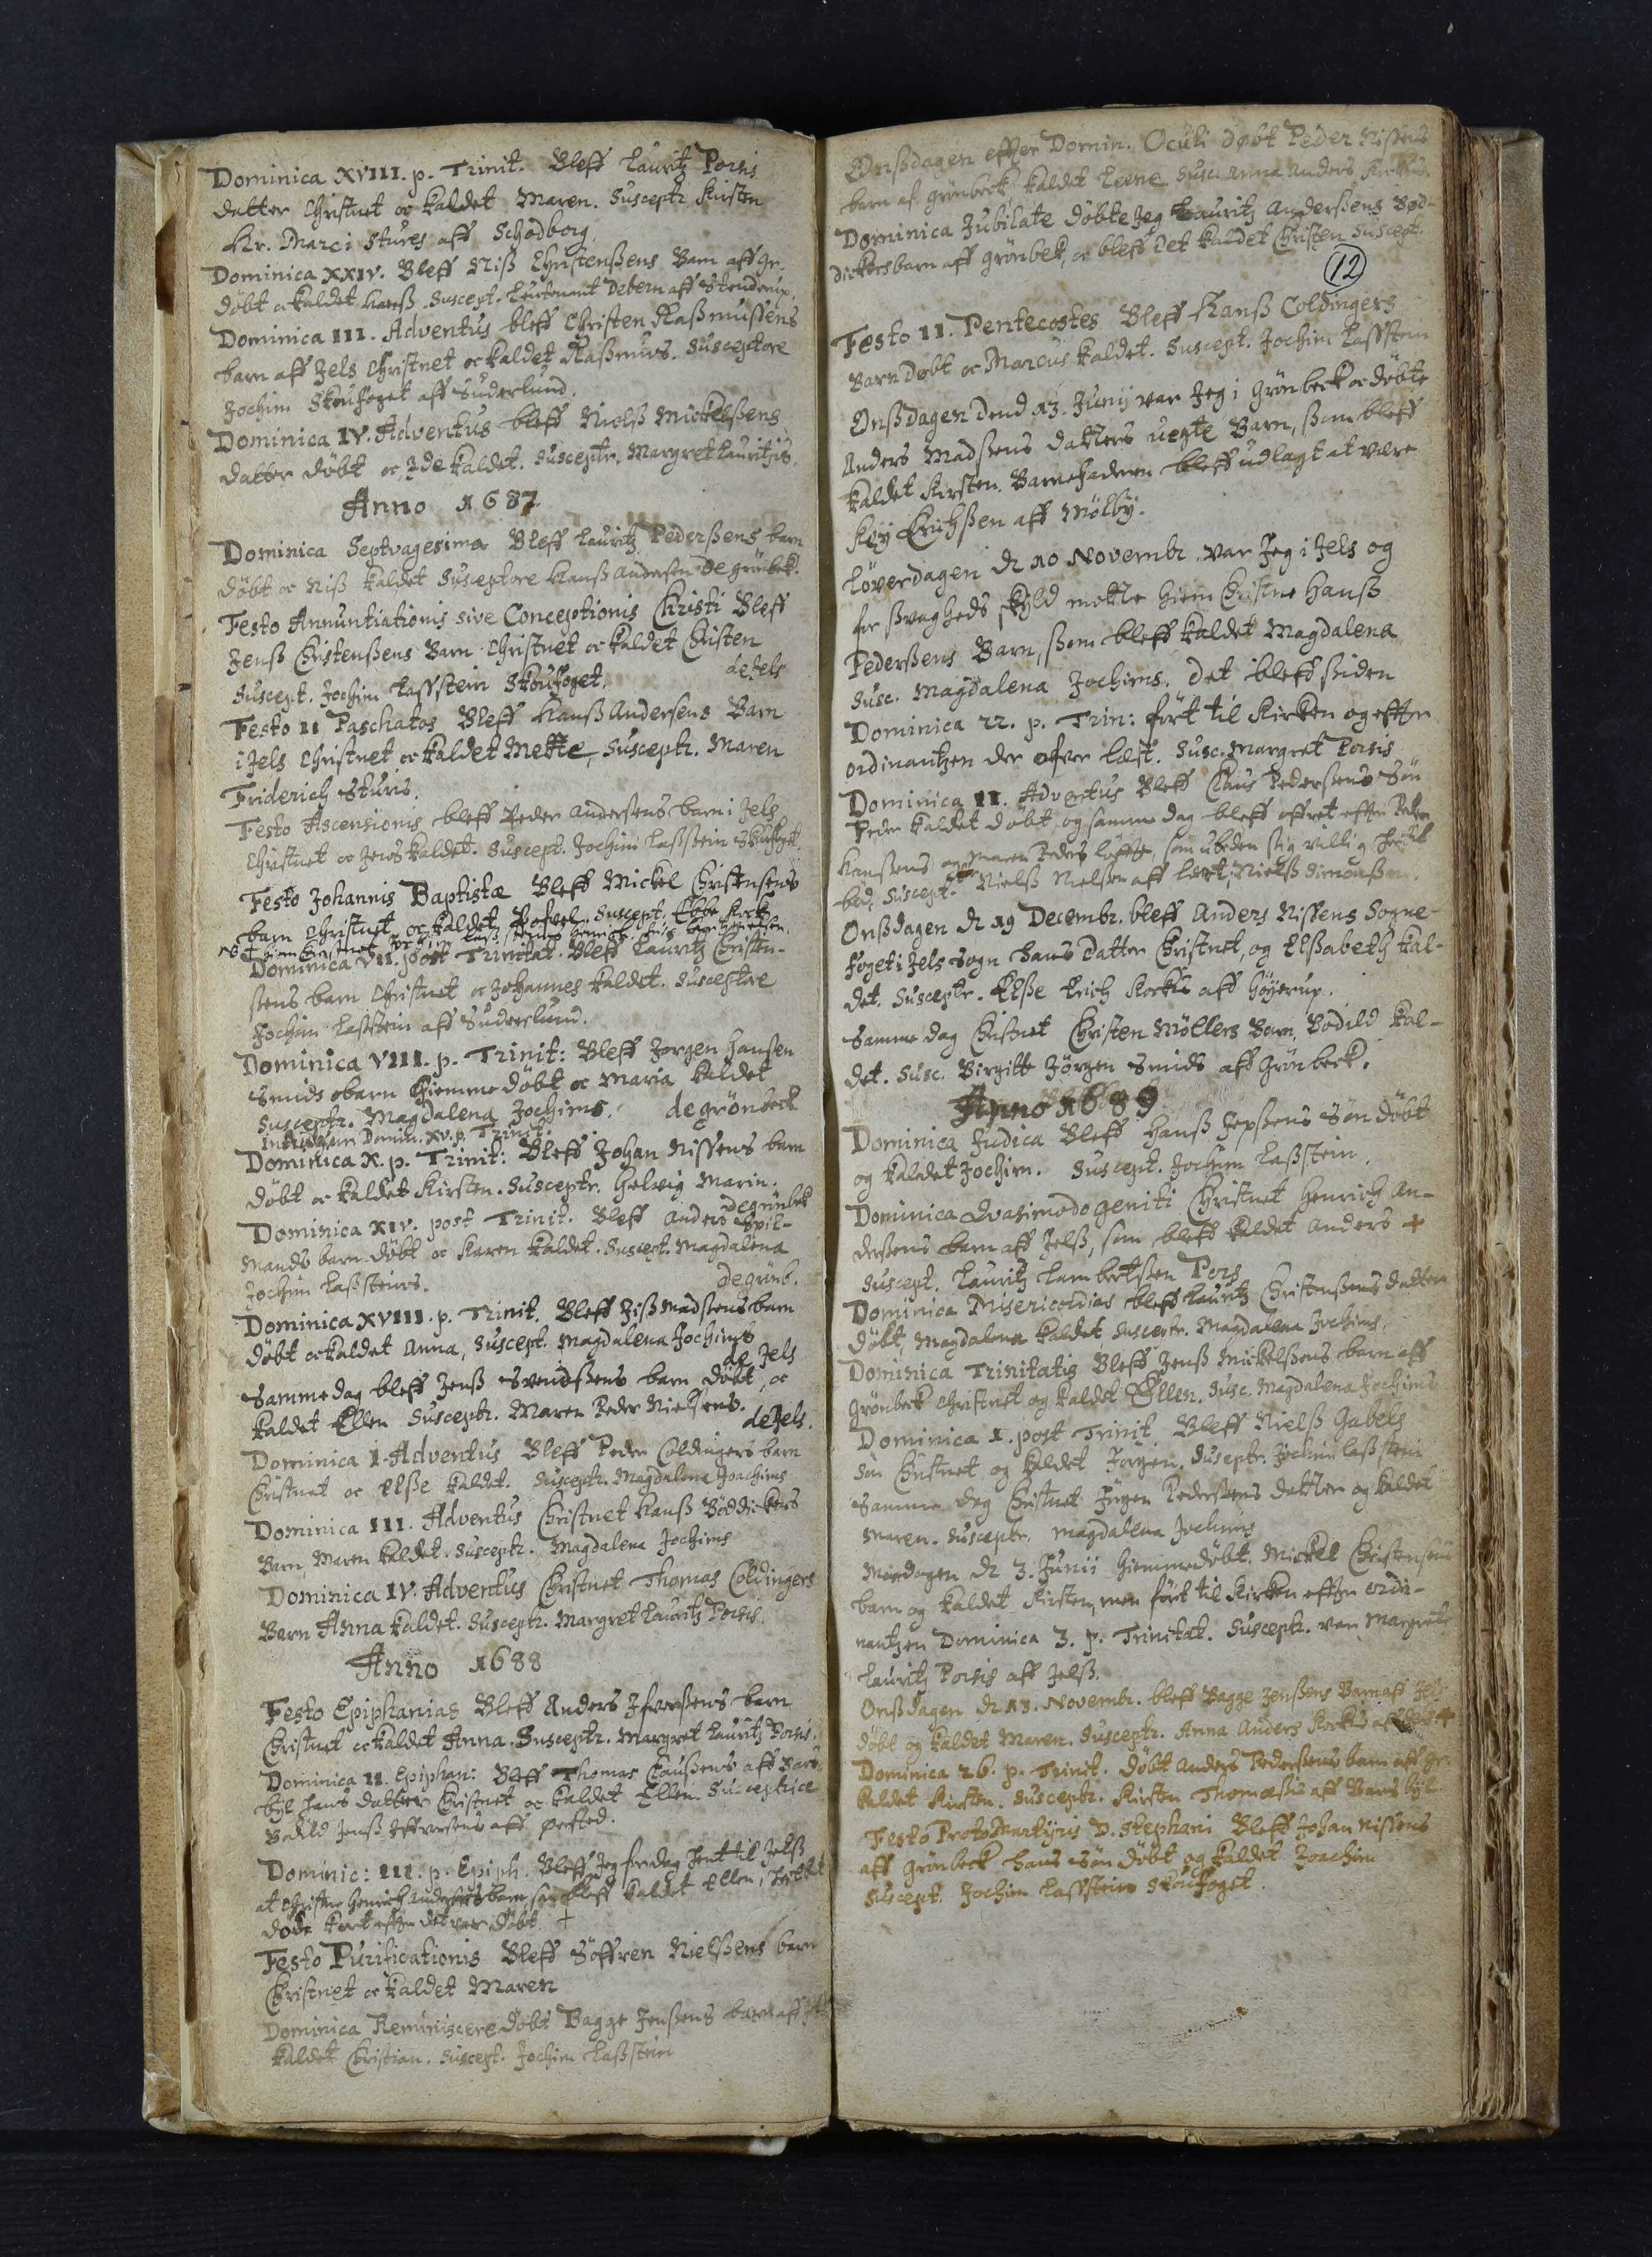Dominica XVIII. p. Trinit. Bleff Lauritz Porsis
datter christnet oc kaldet Maren. Susceptr Kirsten 
HR. Marci Stures aff Schodborg.
Dominica XXIV. Bleff Niss Christensens Barn aff Gr.
døbt oc kaldet Hanss Suscept. Leutnant Debern aff Stenderup.
Dominica III. Adventus bleff Christen Rasmussens
barn aff Jels christnet oc kaldet Rasmus. Susceptore
Jochim Skoufoget aff Suderlund.
Dominica IV. Adventus bleff Niels Mickelssens
datter døbt oc Ide kaldet. Susceptr. Mergret Lauritzis.
Anno 1687.
Dominica Septvagesima Bleff Lauritz Pedersens barn
døbt oc Niss kaldet Susceptore Hans Andersen de Grønbeck.
Festo Annuntiations sive Concesotionis Christi Bleff
Jens Christensens Barn christnet oc kaldet Cristen.
Suscept. Jochim Lasstein Skoufoget.
de Jels
Festo II Paschatos Bleff Hans Andersens Barn
i Jels christnet oc kaldet Mette. Susceptr. Maren
Friderich Sturis.
Festo Ascensionis bleff Peder Andersens barn i Jels
christnet oc Jens kaldet. Suscept. Jochim Lasstein Skoufoget.
Festo Johannis Baptistæ Bleff Mickel Christensens
barn christnet oc kaldet Pofvel. Suscept. Ebbe Kock.
NB + hiemchristnet Jochim Lasstein Gr## ## ## ## ##
Dominica VII post Trinitat. Bleff Lauritz Christen¬
sens barn christnet oc Johannes kaldet. Susceptore
Jochim Lassteins aff Suderlund.
Dominica VIII p. Trinit: Bleff Jørgen Hansen
Smids barn Hiemme Døbt oc Maria kaldet
Susceptr. Magdalena Jochims. de Grønbeck.
in## Domin. XV. p. Trinit.
Dominica X. p. Trinit: Bleff Johan Nissens barn
døbt oc kaldet Kirsten. Susceptr. Helvig Marin.
degrønbeck
Dominica XIV. post Trinit. Bleff Anders Spil¬
mands barn døbt oc Karen kaldet. Suscept. Magdalena
Jochim Lassteins.
De Grønb.
Dominica XVIII. p. Trinit. Bleff Jess Madsens barn
døbt oc kaldet Anna, Suscept. Magdalena Jochims
de Jels
Samme dag bleff Jens Svendsens barn døbt, oc
kaldet Ellen. Susceptr. Maren Peder Nielsens.
de Jels.
Dominica I Adventus Bleff Peder Coldingers barn
Christnet oc Else kaldet. Susceptr. Magdalene Joachims
Dominica III. Adventus Christnet Hanss Bøddickers
barn, Maren kaldet. Susceptr. Magdalene Jochims.
Dominica IV. Adventus Christnet Thomas Coldings
Barn Anna kaldet. Susceptr. Margret Lauritz Porsis.
Anno 1688
Festo Epiphanias Bleff Anders Ifersens barn
Christnet oc kaldet Anna. Susceptr. Margaret Lauritz Porsis.
Dominica II. Epiphan: Bleff Thomas Clausens aff Bars
byl hans datter Christnet oc kaldet Ellen. Susceptrice
Bodild Jenss Iffversens aff Ørsted.
Dominic: III. p. Epiph. Bleff jeg fredag hent til Jelss
at christne Henrich Andersens barn, sa## bleff kaldet Ellen i ha##
døde kort effter det var døbt.
Festo Purificationis Bleff Søffren Nielsens barn
Christnet oc kaldet Maren.
Dominica Reminiscere døbt Bagge Jensens barn aff Jels
kaldet Christian. Suscept. Jochim Lasstein
12
Onsdagen effter Domin. Oculi døbt Peder Nissens
barn af Grønbeck kaldet Leene Susc Anna Anders
Dominica Jubilate døbte jeg Lauritz Andersens Bød¬
dickers barn aff Grønbek, oc bleff det kaldet Christen. Suscept.
Festo II. Pentecostes Bleff Hans Coldingers
Barn døbt oc Marcus Kaldet. Suscept. Jochim Lasstein.
Onsdagen dend 13. Junij var jeg i Grønbeck oc døbte
Anders Madsens datters uegte Barn, som bleff
kaldet Kirsten. Barnefaderen bleff udlagt at være
Key Erichsen aff Mølby.
Løverdagen d 10 Novembr var Jeg i Jels og
for svagheds skyld motte Hiem Cristne Hanss
Pederssens Barn, som bleff kaldet Magdalena
Susc. Magdalena Jochims. Det bleff siden
Dominica 22. p. Trin: ført til kirken og effter
ordinantzen der ofver læst. Susc. Margret Porsis
Dominica II Adventus bleff Claus Pedersens Søn
Peder kaldet døbt og samme dag bleff offret## efter Peder
Hansens og Maren Peders løffte##, som ubden sig villig hertil
bed## Suscept. Niels Nielsen aff Lert, Niels Simonsen.
Onsdagen d 19 Decembr. Bleff Anders Nissens Sogne¬
foget i Jels Sogn hans datter Christnet, og Elssabeth kal¬
det. Susceptr. Elsse Erich Kocks aff Høyrup.
Samme dag Christnet Christen Møllers Barn Bodild kal¬
det. Susc. Birgitt Jørgen Smids aff Grønbeck.
Anno 1689
Dominica Judica Bleff Hans Jepsens Søn døbt
og kaldet Jochim. Suscept. Jochim Lasstein
Dominica Qvasimodogeniti Christnet Henrich An¬
dersens barn aff Jelss, som bleff kaldet Anders.
Suscept. Lauritz Lambretssen Pors
Dominica Misericordias bleff Lauritz Christensens datter
døbt, Magdalena kaldet Susceptr. Magdalena Jochims.
Dominica Trinitatis Bleff Jens Mickelsens barn aff
Grønbeck christnet og kaldet Ellen. Susc. Magdalena Jochims
Dominica I. post Trinit. Bleff Niels Gabels
Søn christnet og kaldet Jørgen. Susceptr. Jochim Lasstein
Samme Dag Christnet Jørgen Pedersens datter og kaldet
Maren. Susceptr. Magdalena Jochims
Mandagen d 3. Junii hiemmedøbt Mickel Christensens
barn og kaldet Kirsten, men ført til Kirken efter ordi¬
nantzen Dominica 3. p. Trinitat. Susceptr. var Margrete
Lauritz Porsis aff Jels.
Onsdagen d 13. Novembr. Bleff Bagge Jensens Barn aff Jels
døbt og kaldet Maren. Susceptr. Anna Anders Kockis af
Dominica 26. p. Trinit. døbt Anders Pedersens barn aff Gr.
kaldet Kirsten. Susceptr. Kirsten Thomasis aff Barsbyl.
Festo Protomartyris S. Stephani Bleff Johan Nissens
aff Grønbeck Hans Søn døbt og kaldet Joachim.
Suscept. Jochim Lasstein Skoufoget.